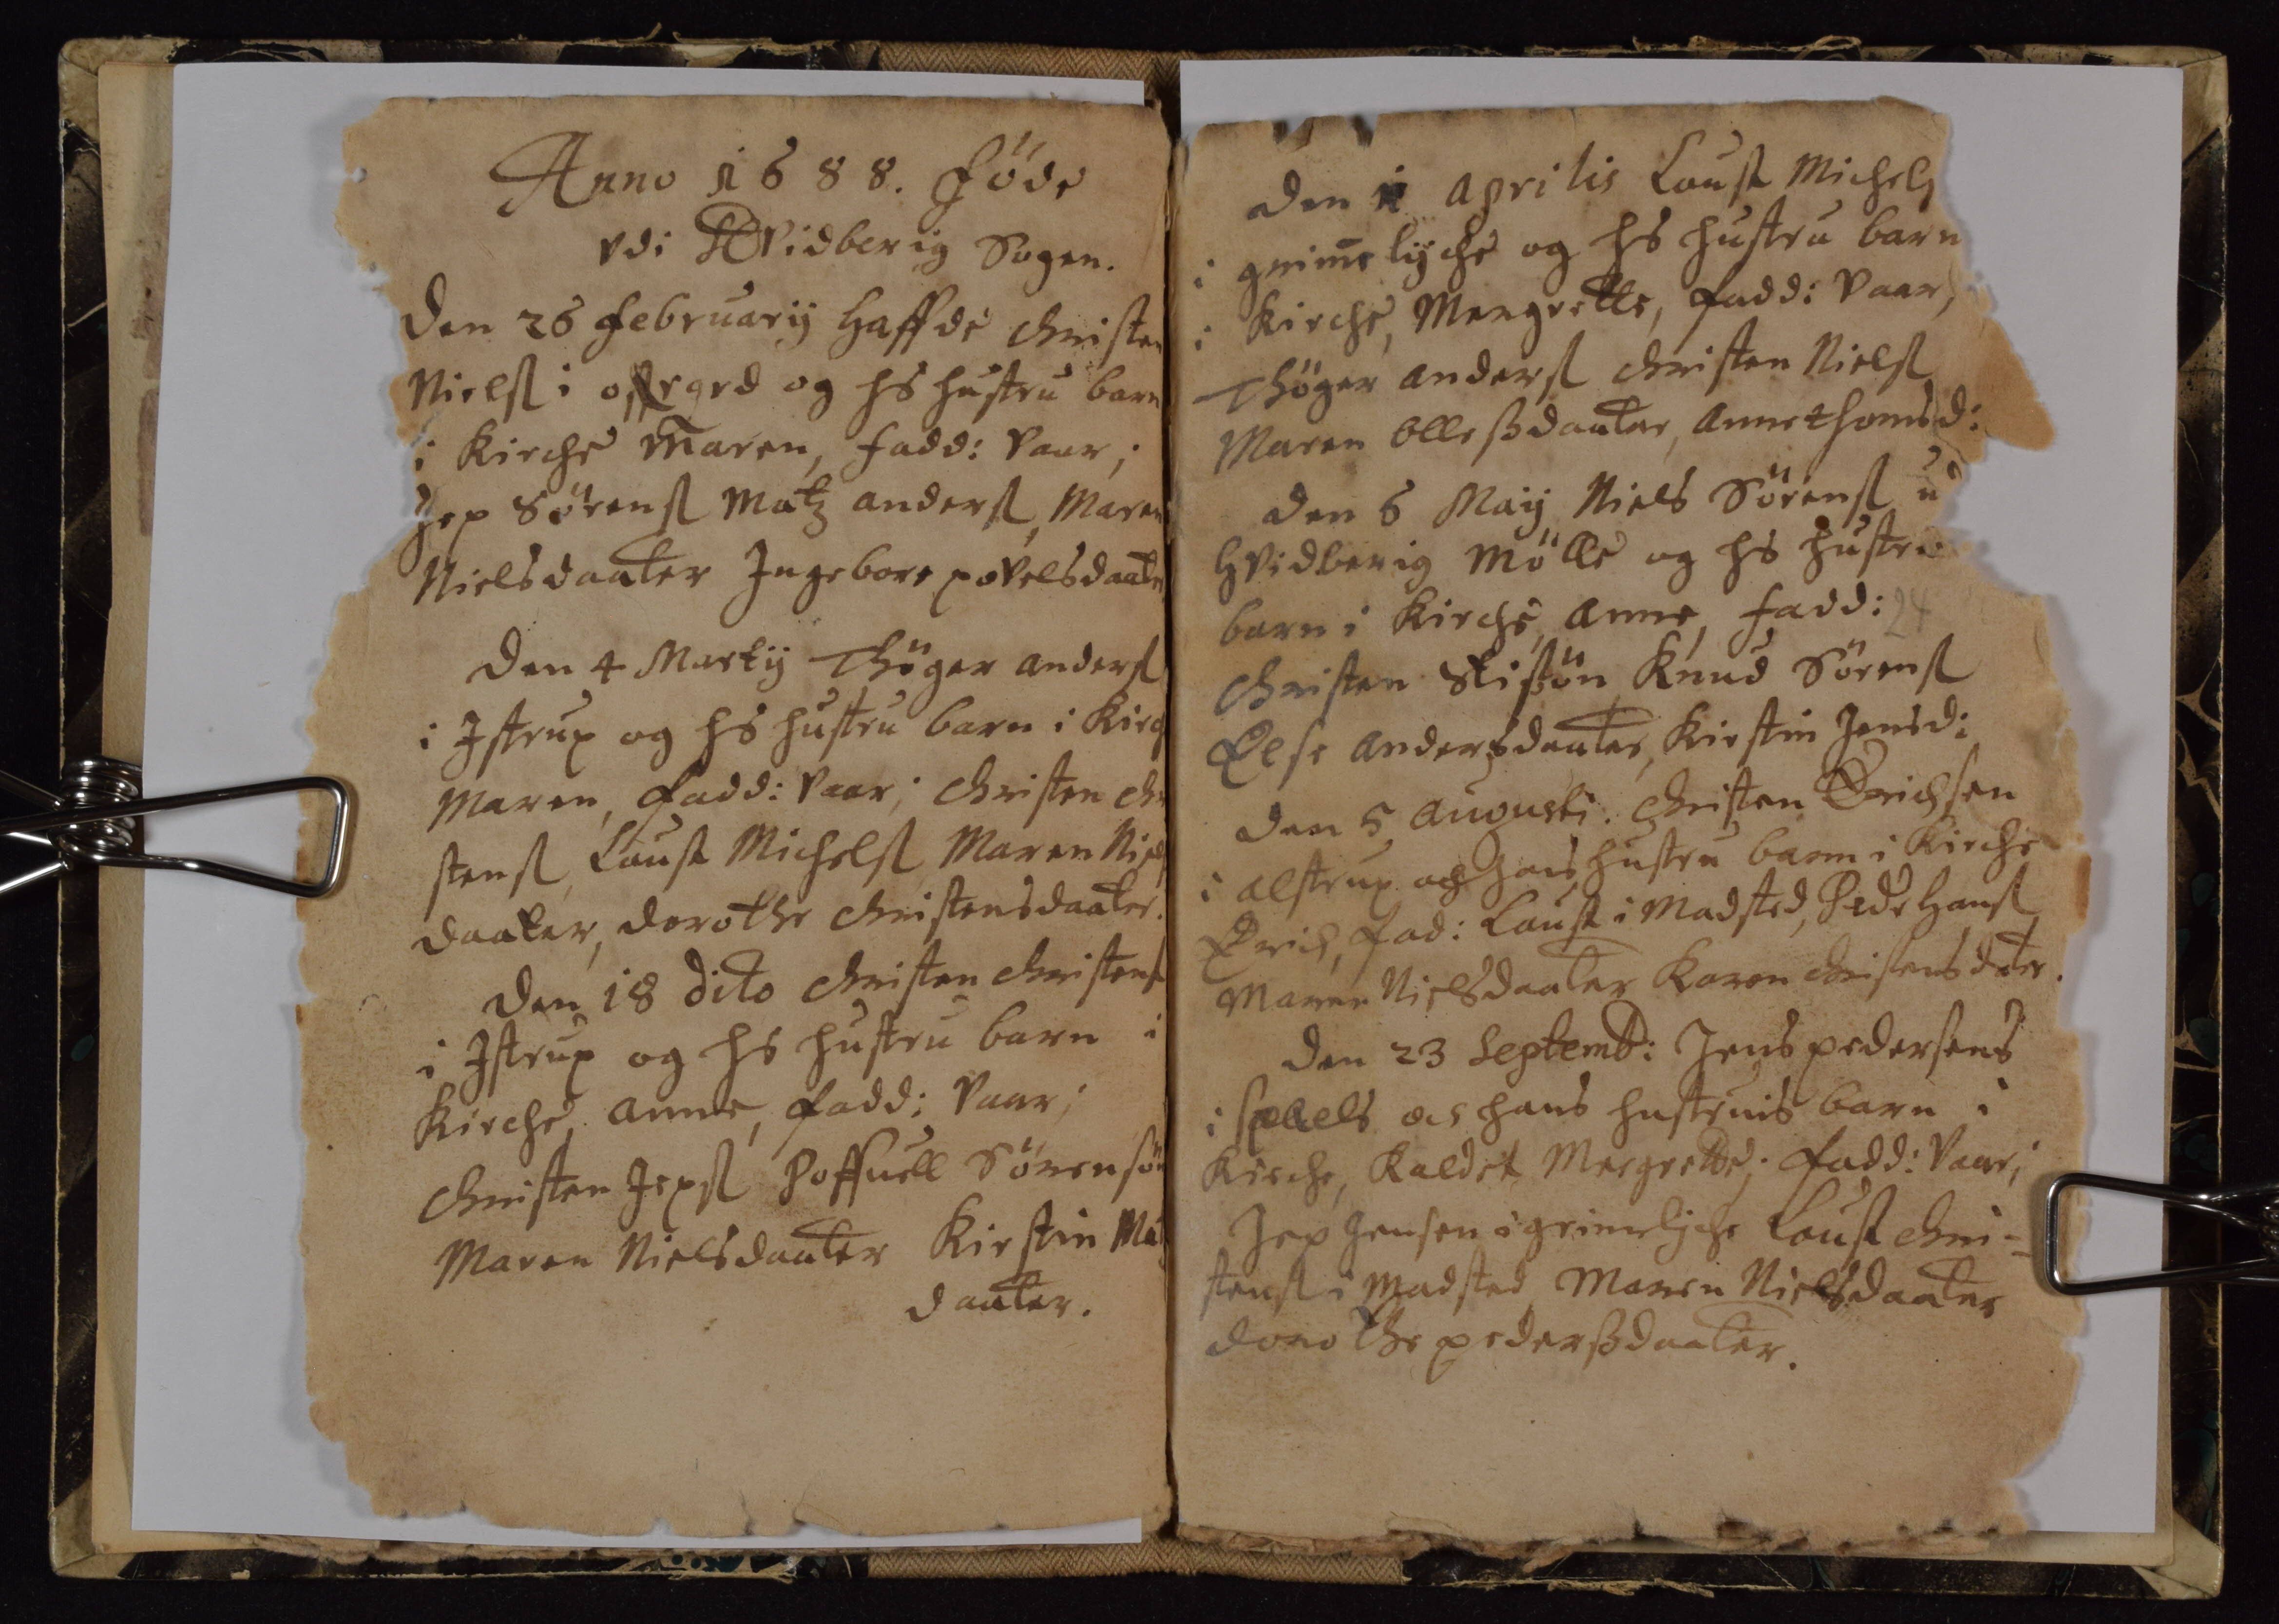Anno 1688. Føde
vdi Hvidberig Sogen.
Den 26 Februarij Haffde christen
Nielsi offrgrd og hs hustru barn
i Kirche Maren, Fadd: vaar;
Jep Sørens Matz Anders Maren
Nielsdaater Ingebore povelsdater
Den 4 Martij Thøger Anders
i Istrup og hs hustru barn i Kirch
Maren, Fadd: vaar, christen Chr
stens, Laust Michels Maren Niels
daater Dorothe christensdaater
Den 18 Dito christen christens
i Istrup og hs hustru barn i
Kirche Anne, Fadd: vaar
christen Jeps Poffuell Sørensøn
Maren Nielsdaater Kirstin Ma
dauter.
Den 1 Aprilis Laust Michels
i Grimmelyche og hs hustru barn
i Kirche Mergrette, Fadd: vaar
Thøger Anders christen Niels
Maren Ollessdaater Anne Thomsd:
den 6 Maij Niels Sørens u
Hvidberig Mølle oghs hustru
barn i Kirche, Anne Fadd:
christen Stisøn Knud Sørens
Else Andersdauter, Kirstin Jensd:
Daa 5 Angustj: christen Erichsen
i Alstrup och hans hustru barn i Kirche
Erich, Fad: Laust i Madsted, Pedr Hans
Maren Nielsdaater Karen christensdater.
Den 23 Septemb: Jens pedersens
i spaels och hans hustru barn i
Kirche, kaldet Mergrette; Fadd: vaar
Jep Jensen i Grimelyche Laust chri¬
stens i Madsted Maren Nielsdaater
Dorothe pederssdaater.
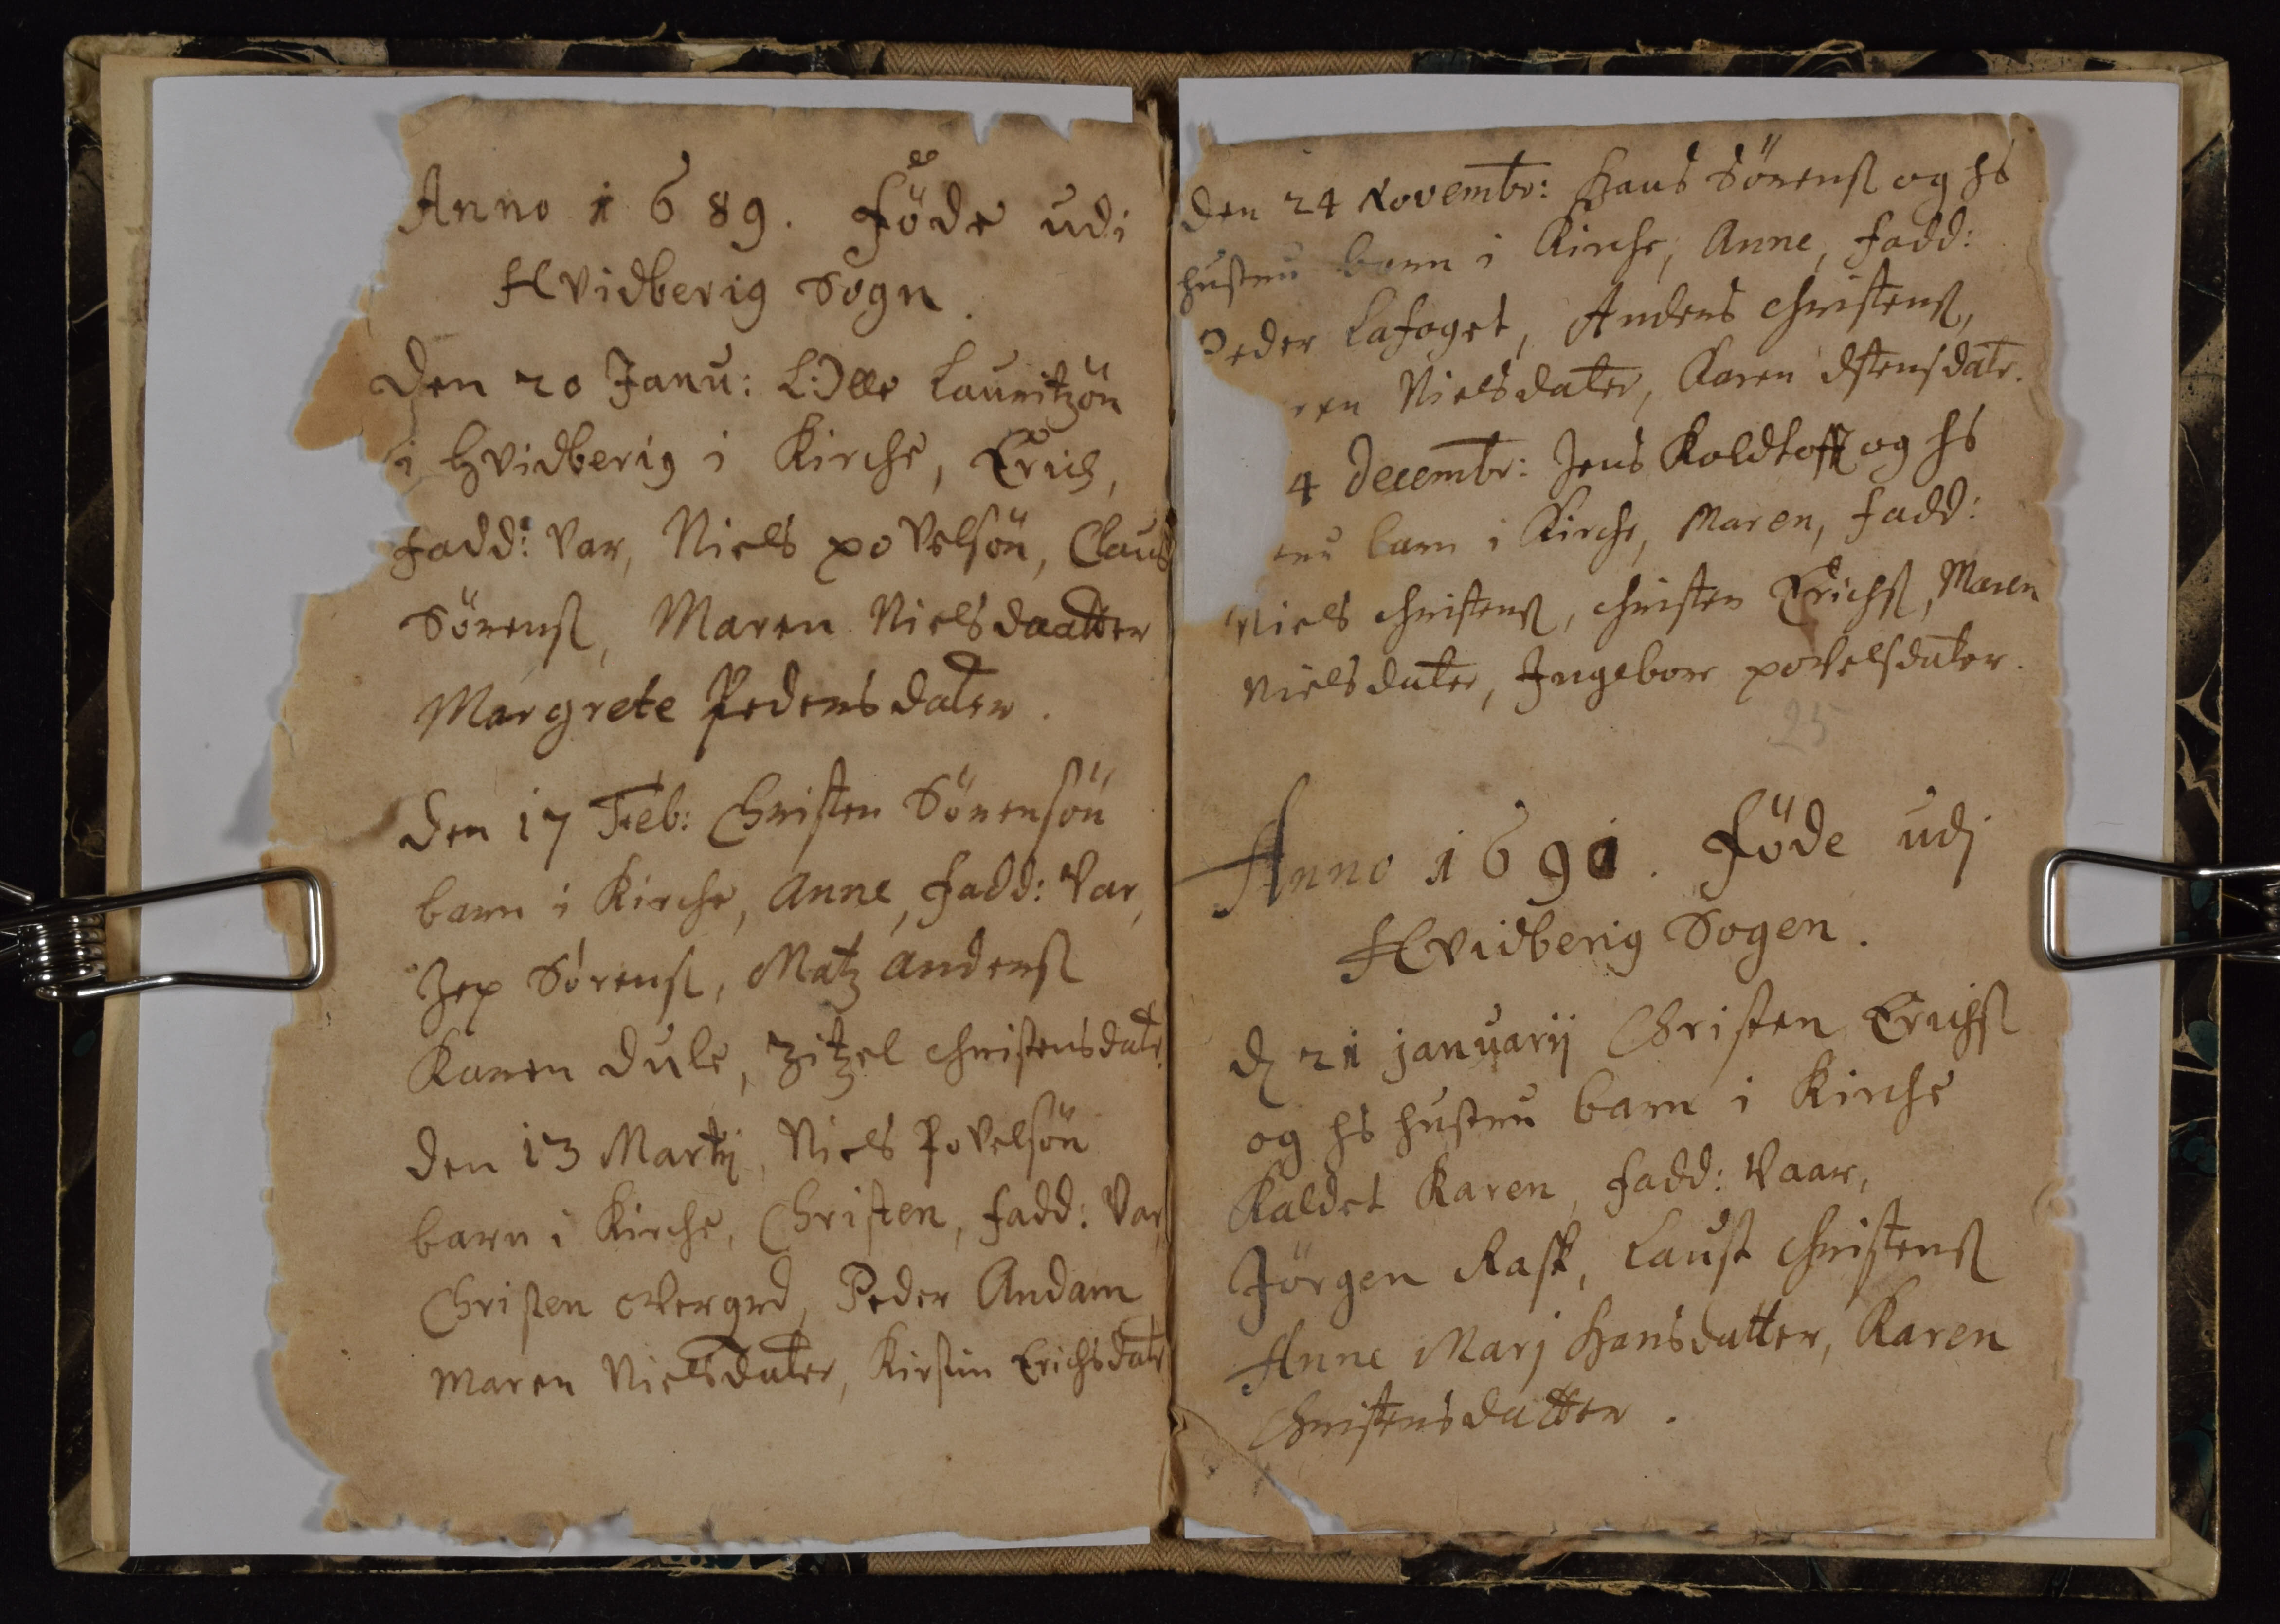Anno 1689. Føde udi
IHvidberig Sogn
Den 20 Janu: Olle Lauritzøn
i Hvidberig i Kirche, Erich,
Fadd: var Niels povelsøn, Claus
Sørens, Maren Nielsdaatter
Margrete Pedersdater
Den 17 Febr: Christen Sørensøn
barn i Kirche, Anne, Fadd: var
Jep Sørens, Matz Anders
Karen Dule, Zitzel Christensdater
Den 13 Martij, Niels Povelsøn
barn i Kirche, Christen, Fadd. var
Christen Overgrd, Peder Andam
Maren Nielsdater, Kirstin Erichsdatr
Den 24 Novembr: Hans Sørens og hs
hustru barn j Kirche, Anne, Fadd:
Peder Lafoget, Anders Christens,
ren Nielsdater, Karen ##tensdatr.
4 Decembr: Jens Koldtoff og hs
tru barn i Kirche, Maren, Fadd:
Niels christens, Christen Erichs, Maren
Nielsdater, Ingebore povelsdater
Anno 1690 Føde udj
Hvidberig Sogen
d 21 Januarij Christen Erichs
og hs hustru barn i Kirche
kaldet Karen Fadd: vaar
Jørgen Rask, Laust Christens
Anne Marj Hansdatter, Karen
Christensdatter